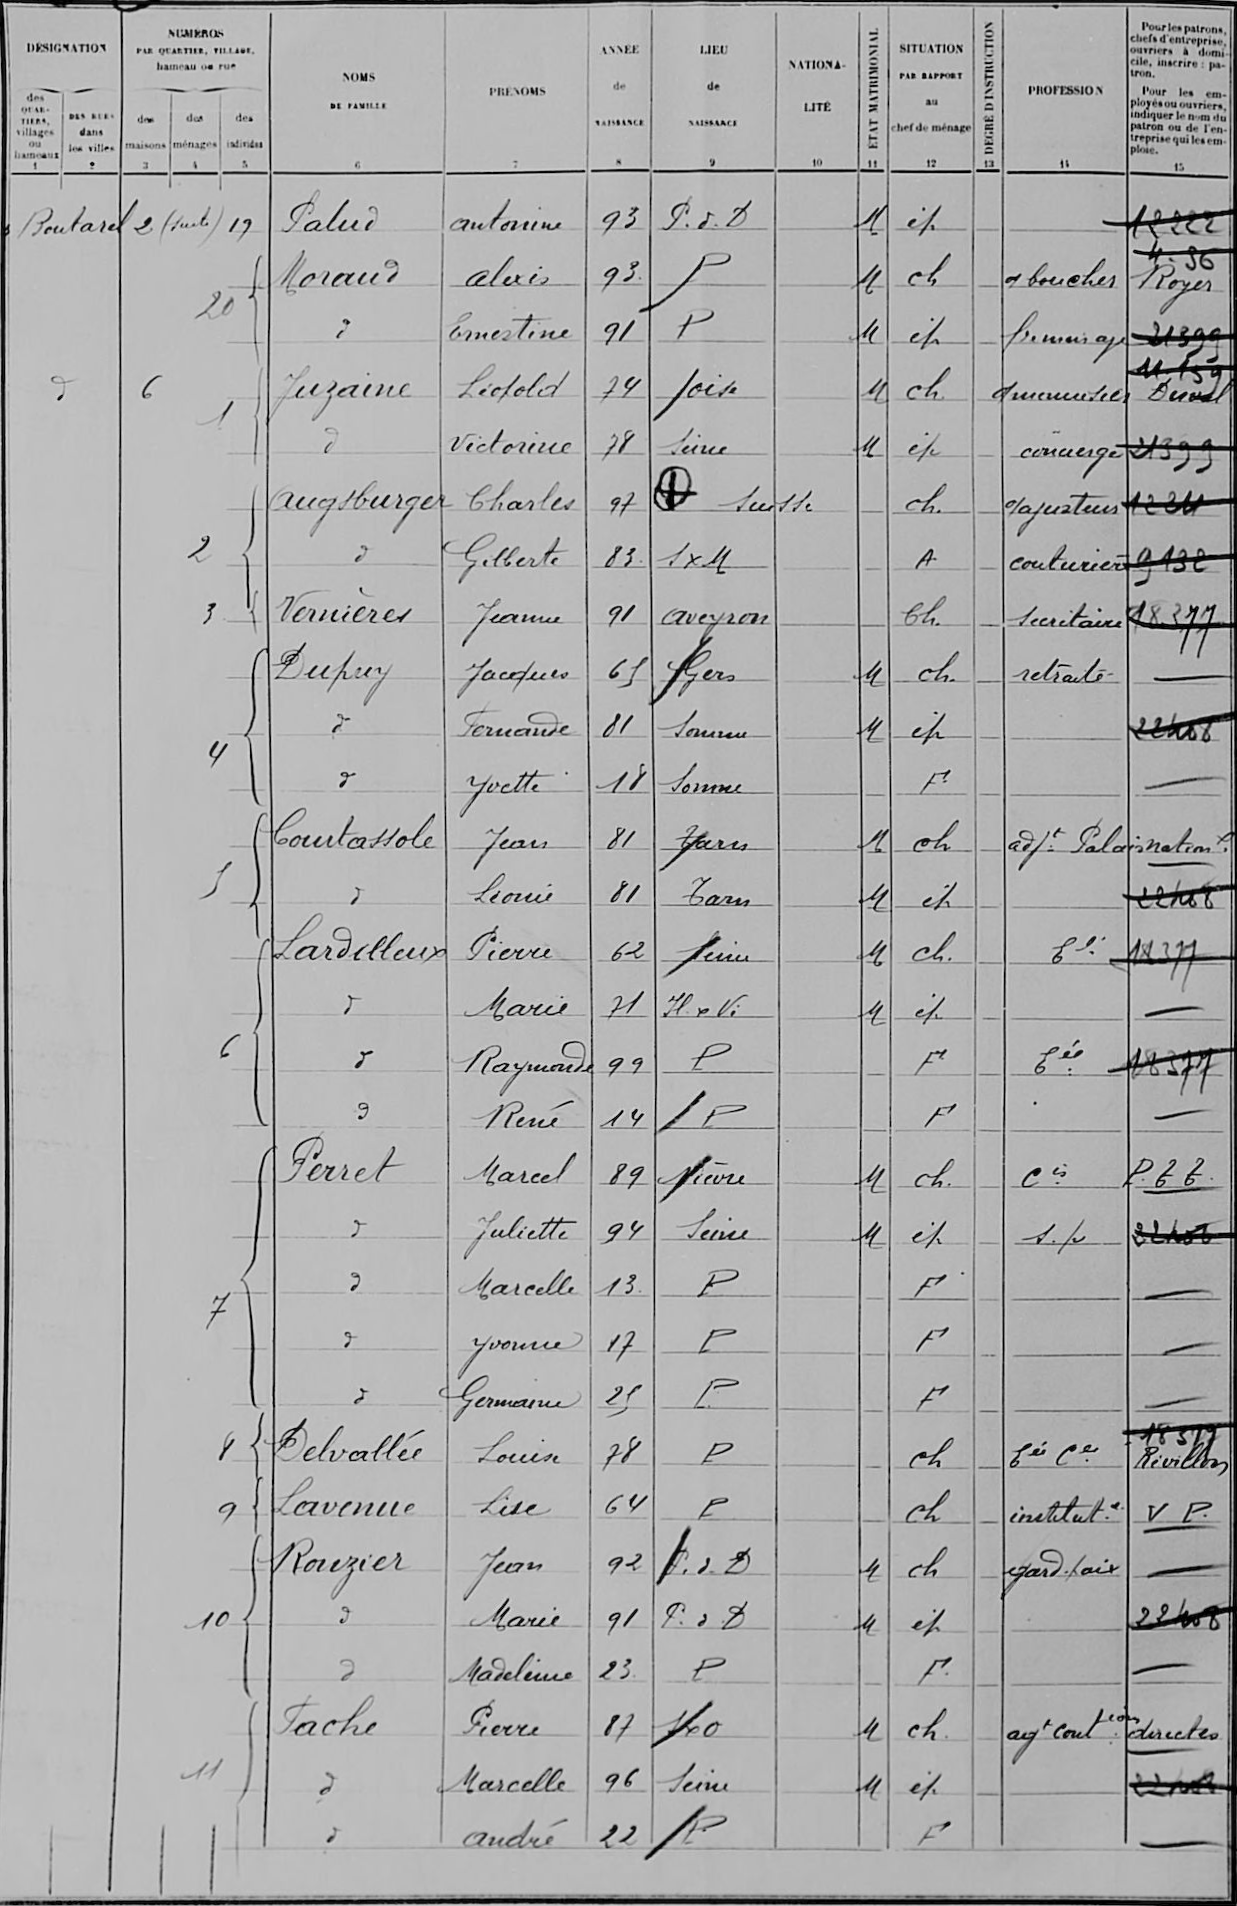Palud/Antonine/93/P d D/¤/M/ép/¤/¤/12222
Moraud/Alexis/93/P/¤/M/ch/¤/o boucher/Royer?436
d/Ernestine/91/P/¤/M/ép/¤/fromager/21399
Juzaine/Léopold/74/Oise/¤/M/ch /¤/o menuisier/Duval?11159
d/Victorine/78/Seine/¤/M/ép/¤/concierge/21399
Augsburger/Charles/97/Suisse/¤/¤/ch /¤/o ajusteur/12211
d/Gilberte/83/S & M/¤/¤/A/¤/couturière/9132
Vernières/Jeanne/91/Aveyron/¤/¤/Ch /¤/Secrétaire/18377
Duprey/Jacques/65/Gers/¤/M/ch /¤/retraite/¤
d°/Fernande/81/Somme/¤/M/ép/¤/¤/22488
d°/Ycette/18/Somme/¤/¤/F/¤/¤/¤
Courtassole/Jean/81/Tarn/¤/M/ch/¤/adjt Palais nation/¤
d/Léonie/81/Tarn/¤/M/ép/¤/¤/22488
Lardilleux/Pierre/62/Seine/¤/M/ch /¤/Eé/18377
d/Marie/71/Il & Vi/¤/M/ép/¤/¤/¤
d/Raymonde/99/P/¤/¤/F/¤/Eée/18377
d/René/14/P/¤/¤/F/¤/¤/¤
Perret/Marcel/89/Nièvre/¤/M/ch /¤/Cis/P T T
d/Juliette/94/Seine/¤/M/ép/¤/S p/22408
d/Marcelle/13/P/¤/¤/F/¤/¤/¤
d/yvonne/17/P/¤/¤/F/¤/¤/¤
d/Germaine/25/P/¤/¤/F/¤/¤/¤
Delvallée/Louise/78/P/¤/¤/ch/¤/Eée Ce/révillon?18377
Lavenue/Lise/64/P/¤/¤/Ch/¤/institutce/V P
Rouzier/Jean/92/P d D/¤/M/ch/¤/gard paix/¤
d/Marie/91/P d D/¤/M/ép/¤/¤/22408
d/Madeleine/3/P/¤/¤/F/¤/¤/¤
Fache/Pierre/87/S & O/¤/M/ch /¤/agt contion directes/¤
d/Marcelle/96/Seine/¤/M/ép/¤/¤/22408
d/André/22/P/¤/¤/F/¤/¤/¤
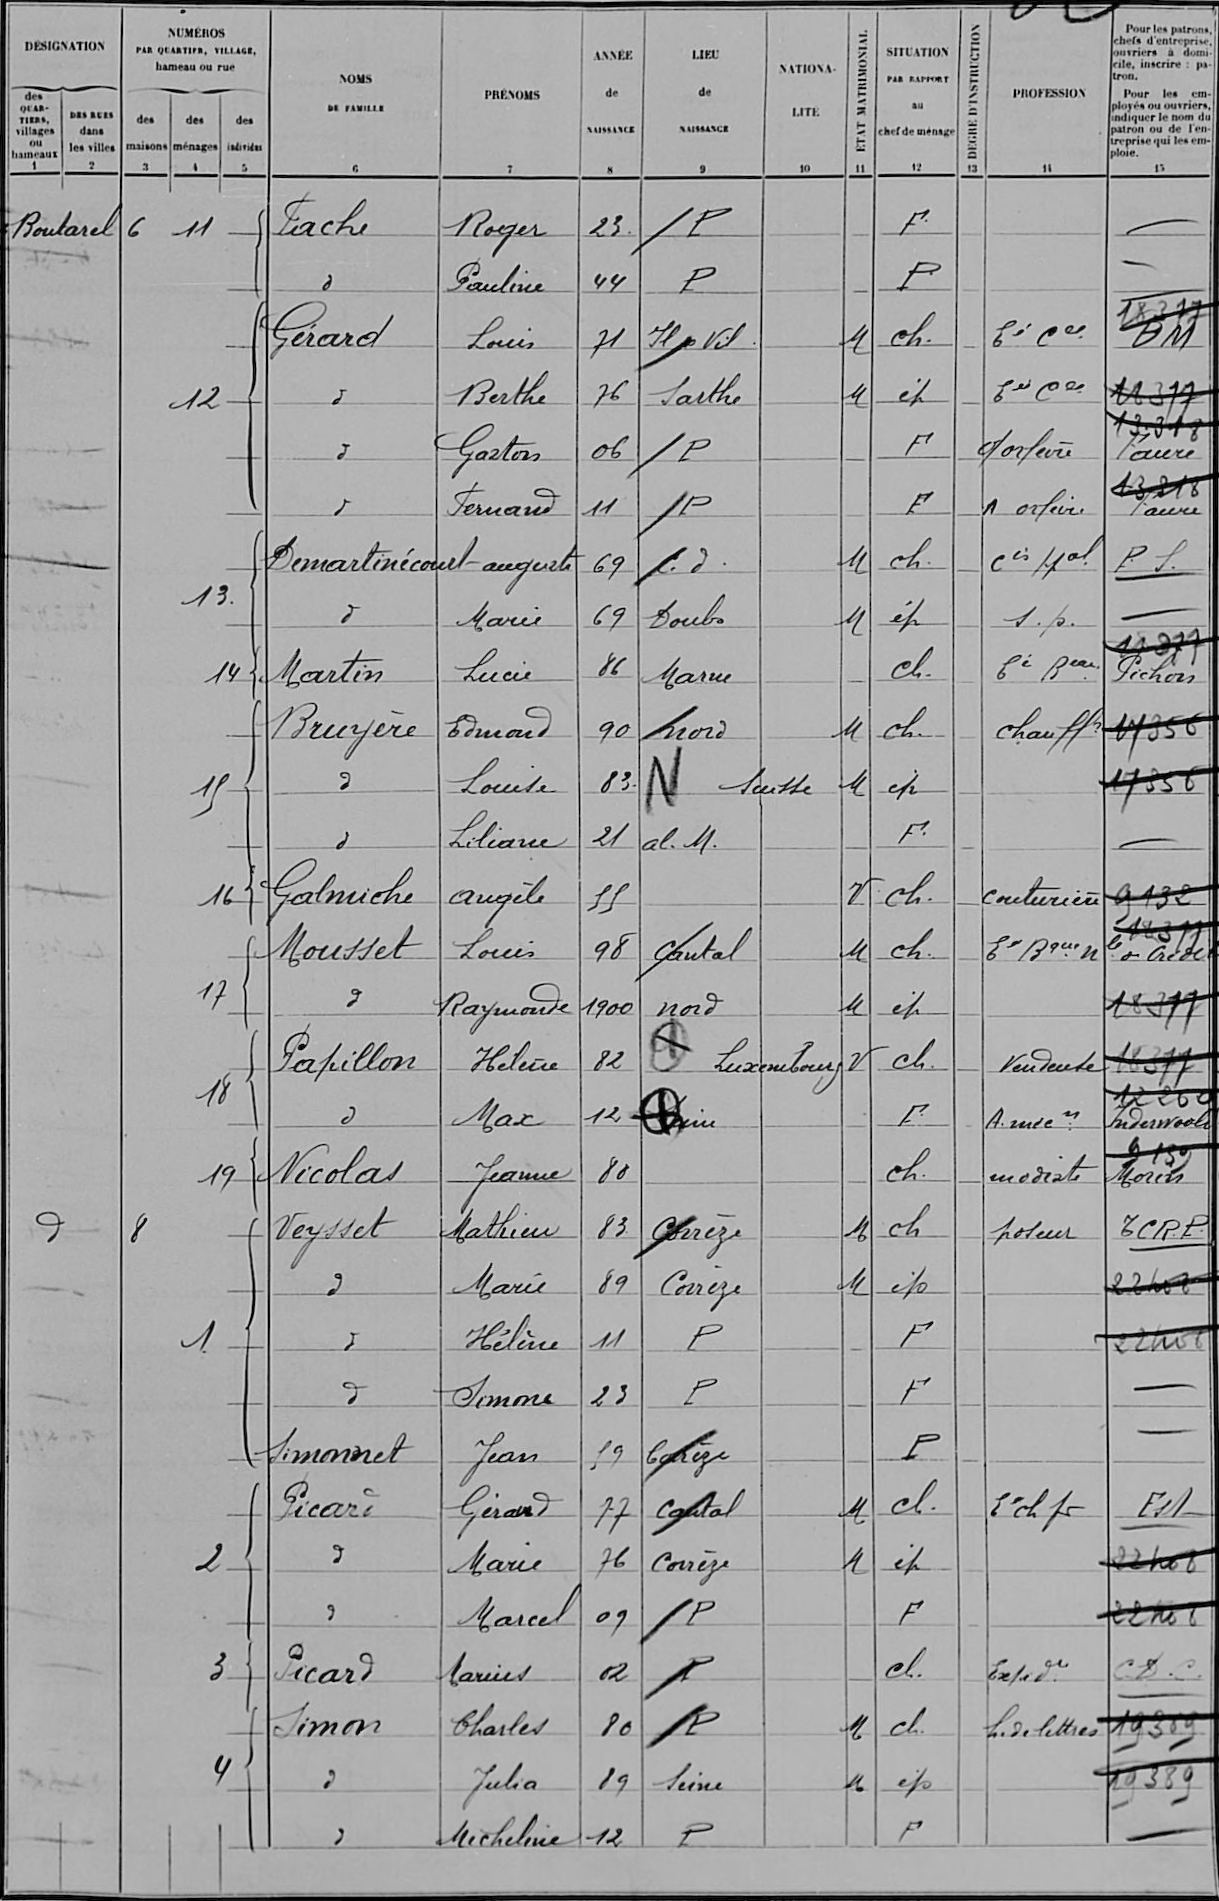Fache/Roger/23/P/¤/¤/F/¤/¤/¤
d/Pauline/4/P/¤/¤/P/¤/¤/¤
Gérard/Louis/71/Il & Vil/¤/M/Ch /¤/Eé Cce/D M?18377
d/Berthe/76/Sarthe/¤/M/ép/¤/Eée Cce/18377
d/Gaston/06/P/¤/¤/F/¤/o orfèvre/Faure?13318
d/Fernand/11/P/¤/¤/F/¤/A orfèvre/Faure13318
Demartinécourt/auguste/69/C d /¤/M/ch /¤/Cis ppal/P S
d/Marie/69/Doubs/¤/M/ép/¤/S p /¤
Martin/Lucie/86/Marne/¤/¤/Ch /¤/Eé Beau/Pichon?18377
Bruyère/Edmond/90/Nord/¤/M/Ch /¤/Chauff/17356
d/Louise/83/Suisse/¤/M/ep/¤/¤/17356
d/Liliane/21/al M /¤/¤/F/¤/¤/¤
Galmiche/Angèle/55/¤/¤/V/ch /¤/couturière/9132
Mousset/Louis/98/Cantal/¤/M/ch /¤/Eé Bqu Nle & Crédit/?18377
d/Raymonde/1900/Nord/¤/M/ép/¤/¤/18377
Papillon/Hélène/82/Luxembourg/¤/V/ch /¤/Vendeuse/18377
d/Max/12/Seine/¤/¤/F/¤/A ruée/Inderwool?12260
Nicolas/Jeanne/88/¤/¤/¤/ch /¤/modiste/Morin?9139
Veysset/Mathieu/83/Corrèze/¤/M/ch/¤/poseur/TCRP
d/Marie/89/Corrèze/¤/M/ép/¤/¤/22408
d/Hélène/11/P/¤/¤/F/¤/¤/22408
d/Simone/23/P/¤/¤/F/¤/¤/¤
Simonnet/Jean/59/Corrèze/¤/¤/P/¤/¤/¤
Picard/Géraud/77/Cantal/¤/M/Ch /¤/Eé ch fe/Est
d/Marie/76/Corrèze/¤/M/ép/¤/¤/22408
d/Marcel/09/P/¤/¤/F/¤/¤/22488
Picard/Marius/02/P/¤/¤/ch /¤/Expedr/CDC
Simon/Charles/80/P/¤/M/Ch /¤/h de lettres/19389
d/Julia/89/Seine/¤/M/ép/¤/¤/19389
d/Micheline/12/P/¤/¤/F/¤/¤/¤